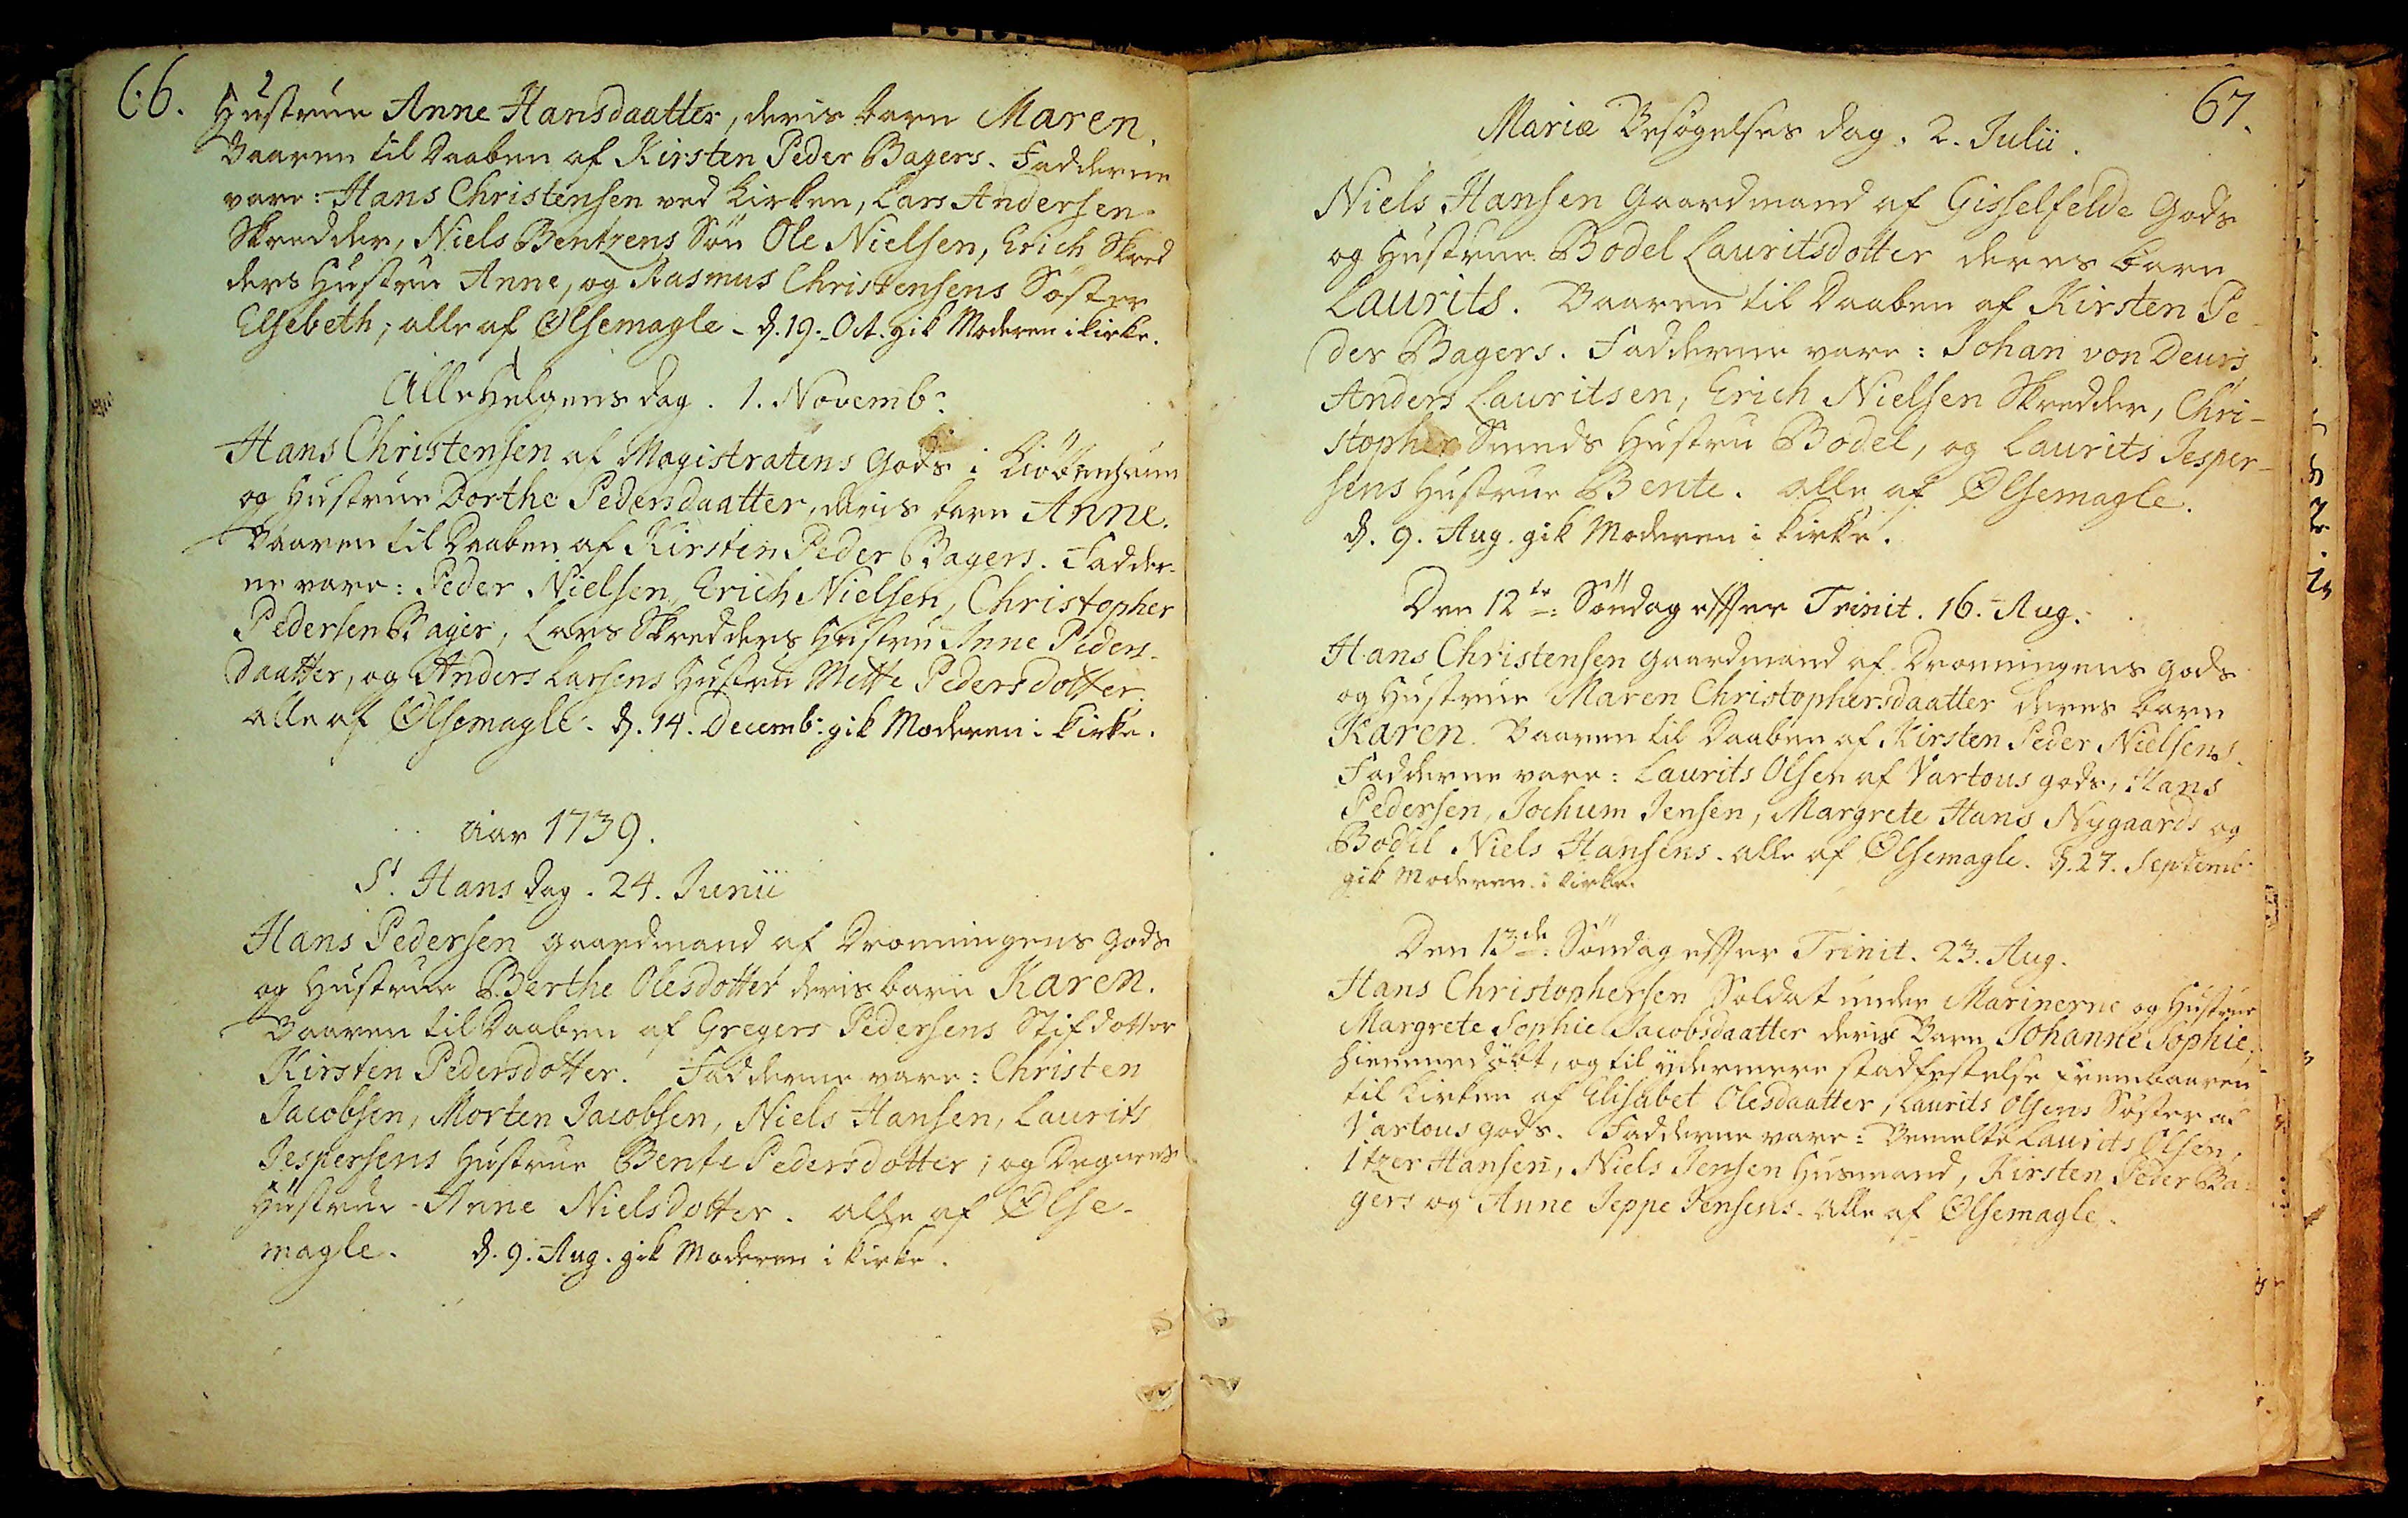66.
Hustrue Anne Hansdaatter, deris Barn Maren.
Baaren til Daaben af Kirsten Peder Bagers. Fadderne
vare: Hans Christensen ved kirken, Lars Andersen.
Skredder, Niels Bentzens Søn Ole Nielsen, Erich Skred
ders Hustrue Anne, og Rasmus Christensens Søster
Elsebeth; alle af Ølsemagle. d.19.Oct. gik Moderen i Kirke
Alle Helgensdag. 1. Novembr:
Hans Christensen af Magistratens Gods i Kiøbenhaun
og Hustrue Dorthe Pedersdaatter, deris Barn Anne.
Baaren til Daaben af Kirsten Peder Bagers. Fadder¬
ne vare: Peder Nielsen, Erich Nielsen, Christopher
Pedersen Bager, Lars Skredders Hustrue Anne Peders¬
daatter, og Anders Larsens Hustrue Mette Pedersdaatter.
Alle af Ølsemagle. d. 14.Decembr: gik Moderen i Kirke.
Aar 1739.
St. Hans Dag. 24. Junii
Hans Pedersen Gaardmand af Dronningens Gods
og Hustrue Berthe Olesdotter deris Barn Karen.
Baaren til Daaben af Gregers Pedersens Stifdotter
Kirsten Pedersdotter. Fadderne vare: Christen
Jacobsen, Morten Jacobsen, Niels Hansen, Laurits
Jespersens Hustrue Berte Pedersdatter; og Degnens
Hustrue Anne Nielsdotter. Alle af Ølse¬
magle. d.9.Aug. gik Moderen i Kirke.
67.
Mariæ Besøgelses Dag. 2. Julii.
Niels Hansen Gaardmand af Gisselfelde Gods
og Hustrue Bodel Lauritsdatter deris Barn
Laurits. Baaren til Daaben af Kirsten Pe
der Bagers. Fadderne vare: Johan von Deurs,
Anders Lauritsen, Erich Nielsen Skredder, Chri¬
stopher Smeds Hustrue Bodel, og Laurits Jesper¬
sens Hustrue Bente. Alle af Ølsemagle.
d. 9. Aug. gik Moderen i Kirke.
Den 12te Søndag efter Trinit. 16. Aug.
Hans Christensen Gaardmand af Dronningens Gods
og Hustrue Maren Christophersdaatter deris Barn
Karen. Baaren til Daaben af Kirsten Peder Nielsens.
Fadderne vare: Laurits Olsen af Vartous Gods, Hans
Pedersen, Jochum Jensen, Margrete Hans Nygaards og
Bodil Niels Hansens. Alle af Ølsemagle. d. 27. Septembr.
gik Moderen i Kirke.
Den 13de: Søndag efter Trinit. 23. Aug.
Hans Christophersen Soldat under Marinerne og Hustrue
Margrete Sophie Jacobsdaatter deris Barn Johanne Sophie.
hiemmedøbt, og til ydermere stadfæstelse frembaaren
til Kirken af Elisabet Olesdaatter, Laurits Olsens Søster af
Vartous Gods. Fadderne vare: Bemelte Laurits Olsen,
Itzer Hansen, Niels Jensen Husmand, Kirsten Peder Ba
gers og Anne Jeppe Jensens. Alle af Ølsemagle.